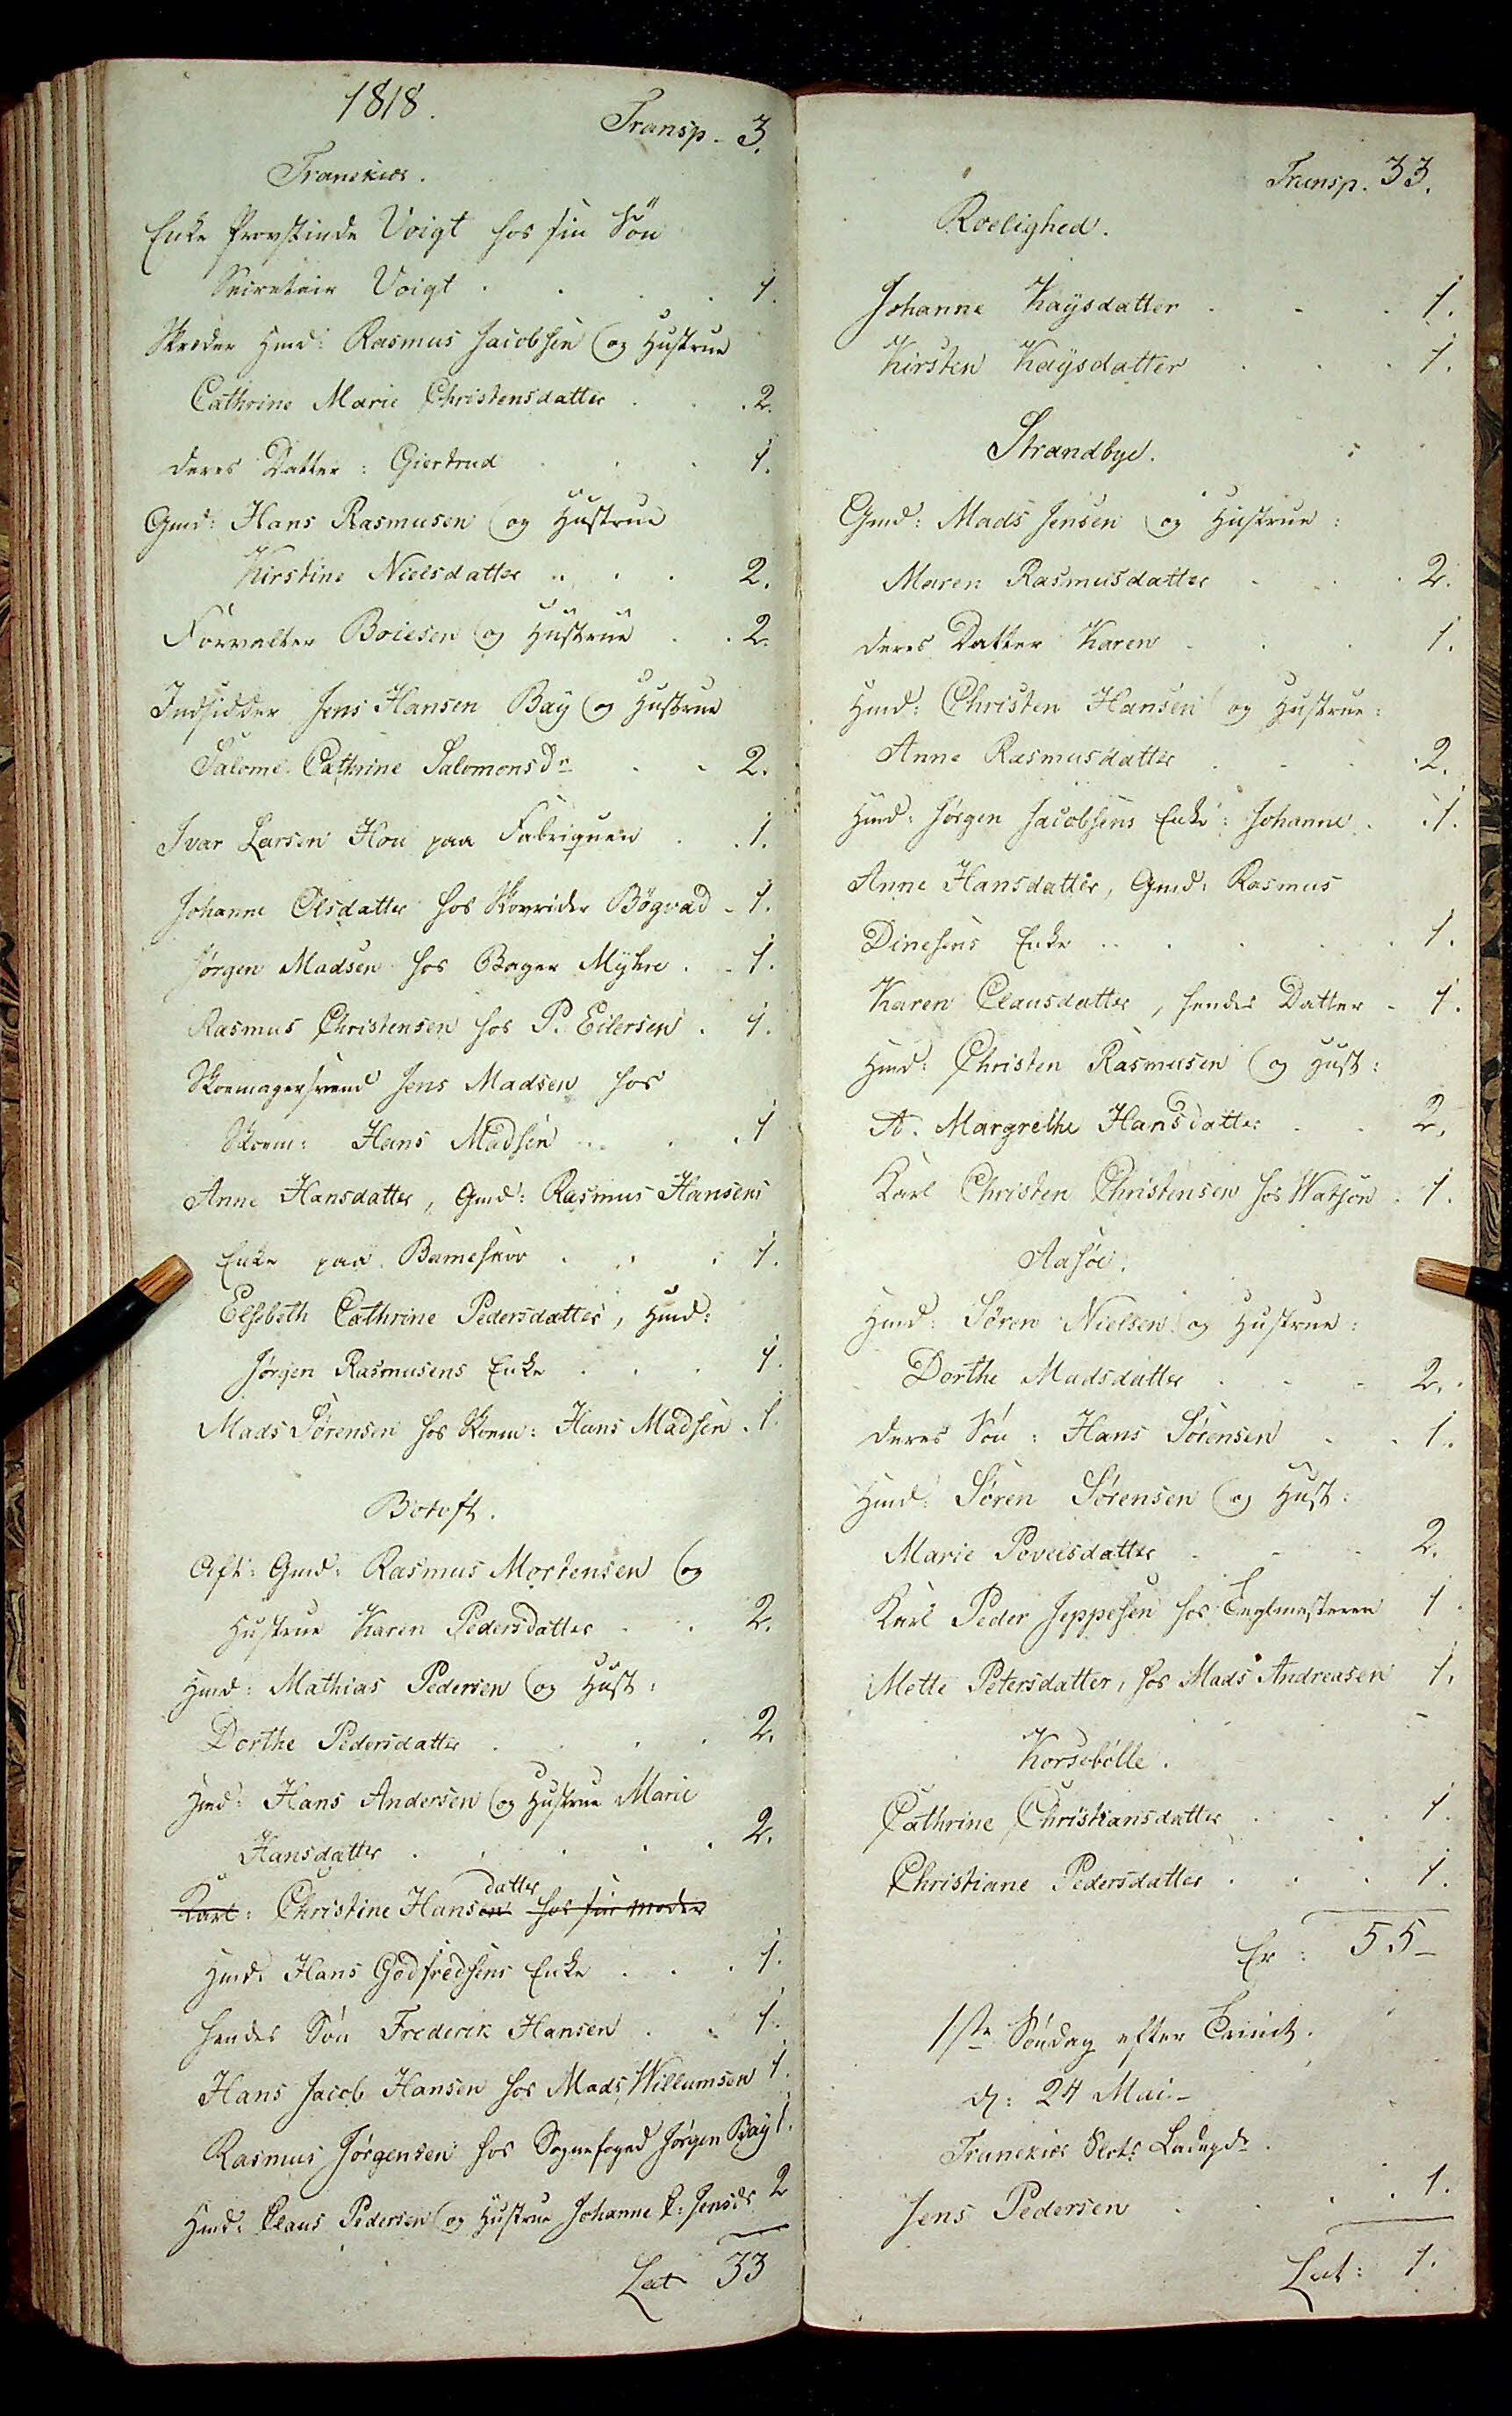1818
Transp - 3.
Tranekiær.
Enke Provstinde Voigt hos sin Søn
Secretair Voigt . . . . 1.
Skreder Hmd: Rasmus Jacobsen og Hustrue
Cathrine Marie Christensdatter . . . 2.
deres Datter: Giertrud . . . 1.
Gmd: Hans Rasmusen og Hustrue
Kirstine Nielsdatter . . . . 2.
Forvalter Boiesen og Hustrue . . 2.
Indsidder Jens Hansen Bay og Hustrue
Salome Cathrine Salomonsdr . .2.
Ivar Larsen Hou paa Fabriquen . . 1.
Johanne Olsdatter hos Skovrider Bøgvad - 1.
Jørgen Madsen hos Bagen Myhre . . 1.
Rasmus Christensen hos P. Eilersen . 1.
Skoemagersvend Jens Madsen hos
Skoem: Hans Madsen . . . 1
Anne Hansdatter, Gmd: Rasmus Hansens
Enke paa Bameskov . . . 1.
Elsebeth Cathrine Pedersdatter, Hmd:
Jørgen Rasmusens Enke . . . 1.
Mads Sørensen hos Skoem: Hans Madsen . 1.
Botoft.
Aft: Gmd: Rasmus Mortensen og
Hustrue Karen Pedersdatter . . 2.
Hmd: Mathias Pedersen og Hust:
Dorthe Pedersdatter . . . . 2.
Hmd: Hans Andersen og Hustrue Marie
Hansdatter . . . . . 2.
datter
Karl: Christine Hansen hos sin Morder
Hmd: Hans Godfredsens Enke . . . 1.
hendes Søn Frederik Hansen . . 1.
Hans Jacob Hansen hos Mads Willumsen 1.
Rasmus Jørgensen hos Sognefoged Jørgen Bay 1.
Hmd: Claus Pedersen og Hustrue Johanne C: Jensdr 2
Lat 33
Transp. 33.
Roelighed.
Johanne Kaysdatter . . . 1.
Kirsten Kaysdatter . . . 1.
Srrandbye.
Gmd: Mads Jensen og Hustrue:
Maren Rasmusdatter . . . 2.
deres Datter Karen . . . 1.
Hmd: Christen Hansen og Hustrue
Anne Rasmusdatter . . . . 2.
Hmd: Jørgen Jacobsens Enke: Johanne . . 1.
Anne Hansdatter, Gmd: Rasmus
Dinesens Enke . . . . . . 1.
Karen Clausdatter, hendes Datter . 1.
Hmd: Christen Rasmusen og Hust:
A. Margrethe Hansdatter . . 2.
Karl Christen Christensen hos Watson . 1.
Aasøe.
Hmd: Søren Nielsen og Hustrue:
Dorthe Madsdatter . . . 2.
deres Søn: Hans Sørensen . . 1.
Hmd: Søren Sørrensen og Hust:
Marie Povelsdatter . . . 2.
Karl Peder Jeppesen hos Teglmesteren 1.
Mette Pedersdatter, hos Hans Andreasen 1.
Korsebølle.
Cathrine Christiansdatter . . . 1.
Christiane Pedersdatter . . . 1.
Er: 55-
1ste Søndag efter Trinit.
d: 24 Mai-
Tranekiær Slots Ladegdr.
Jens Pedersen . . . . 1.
Lat: 1.
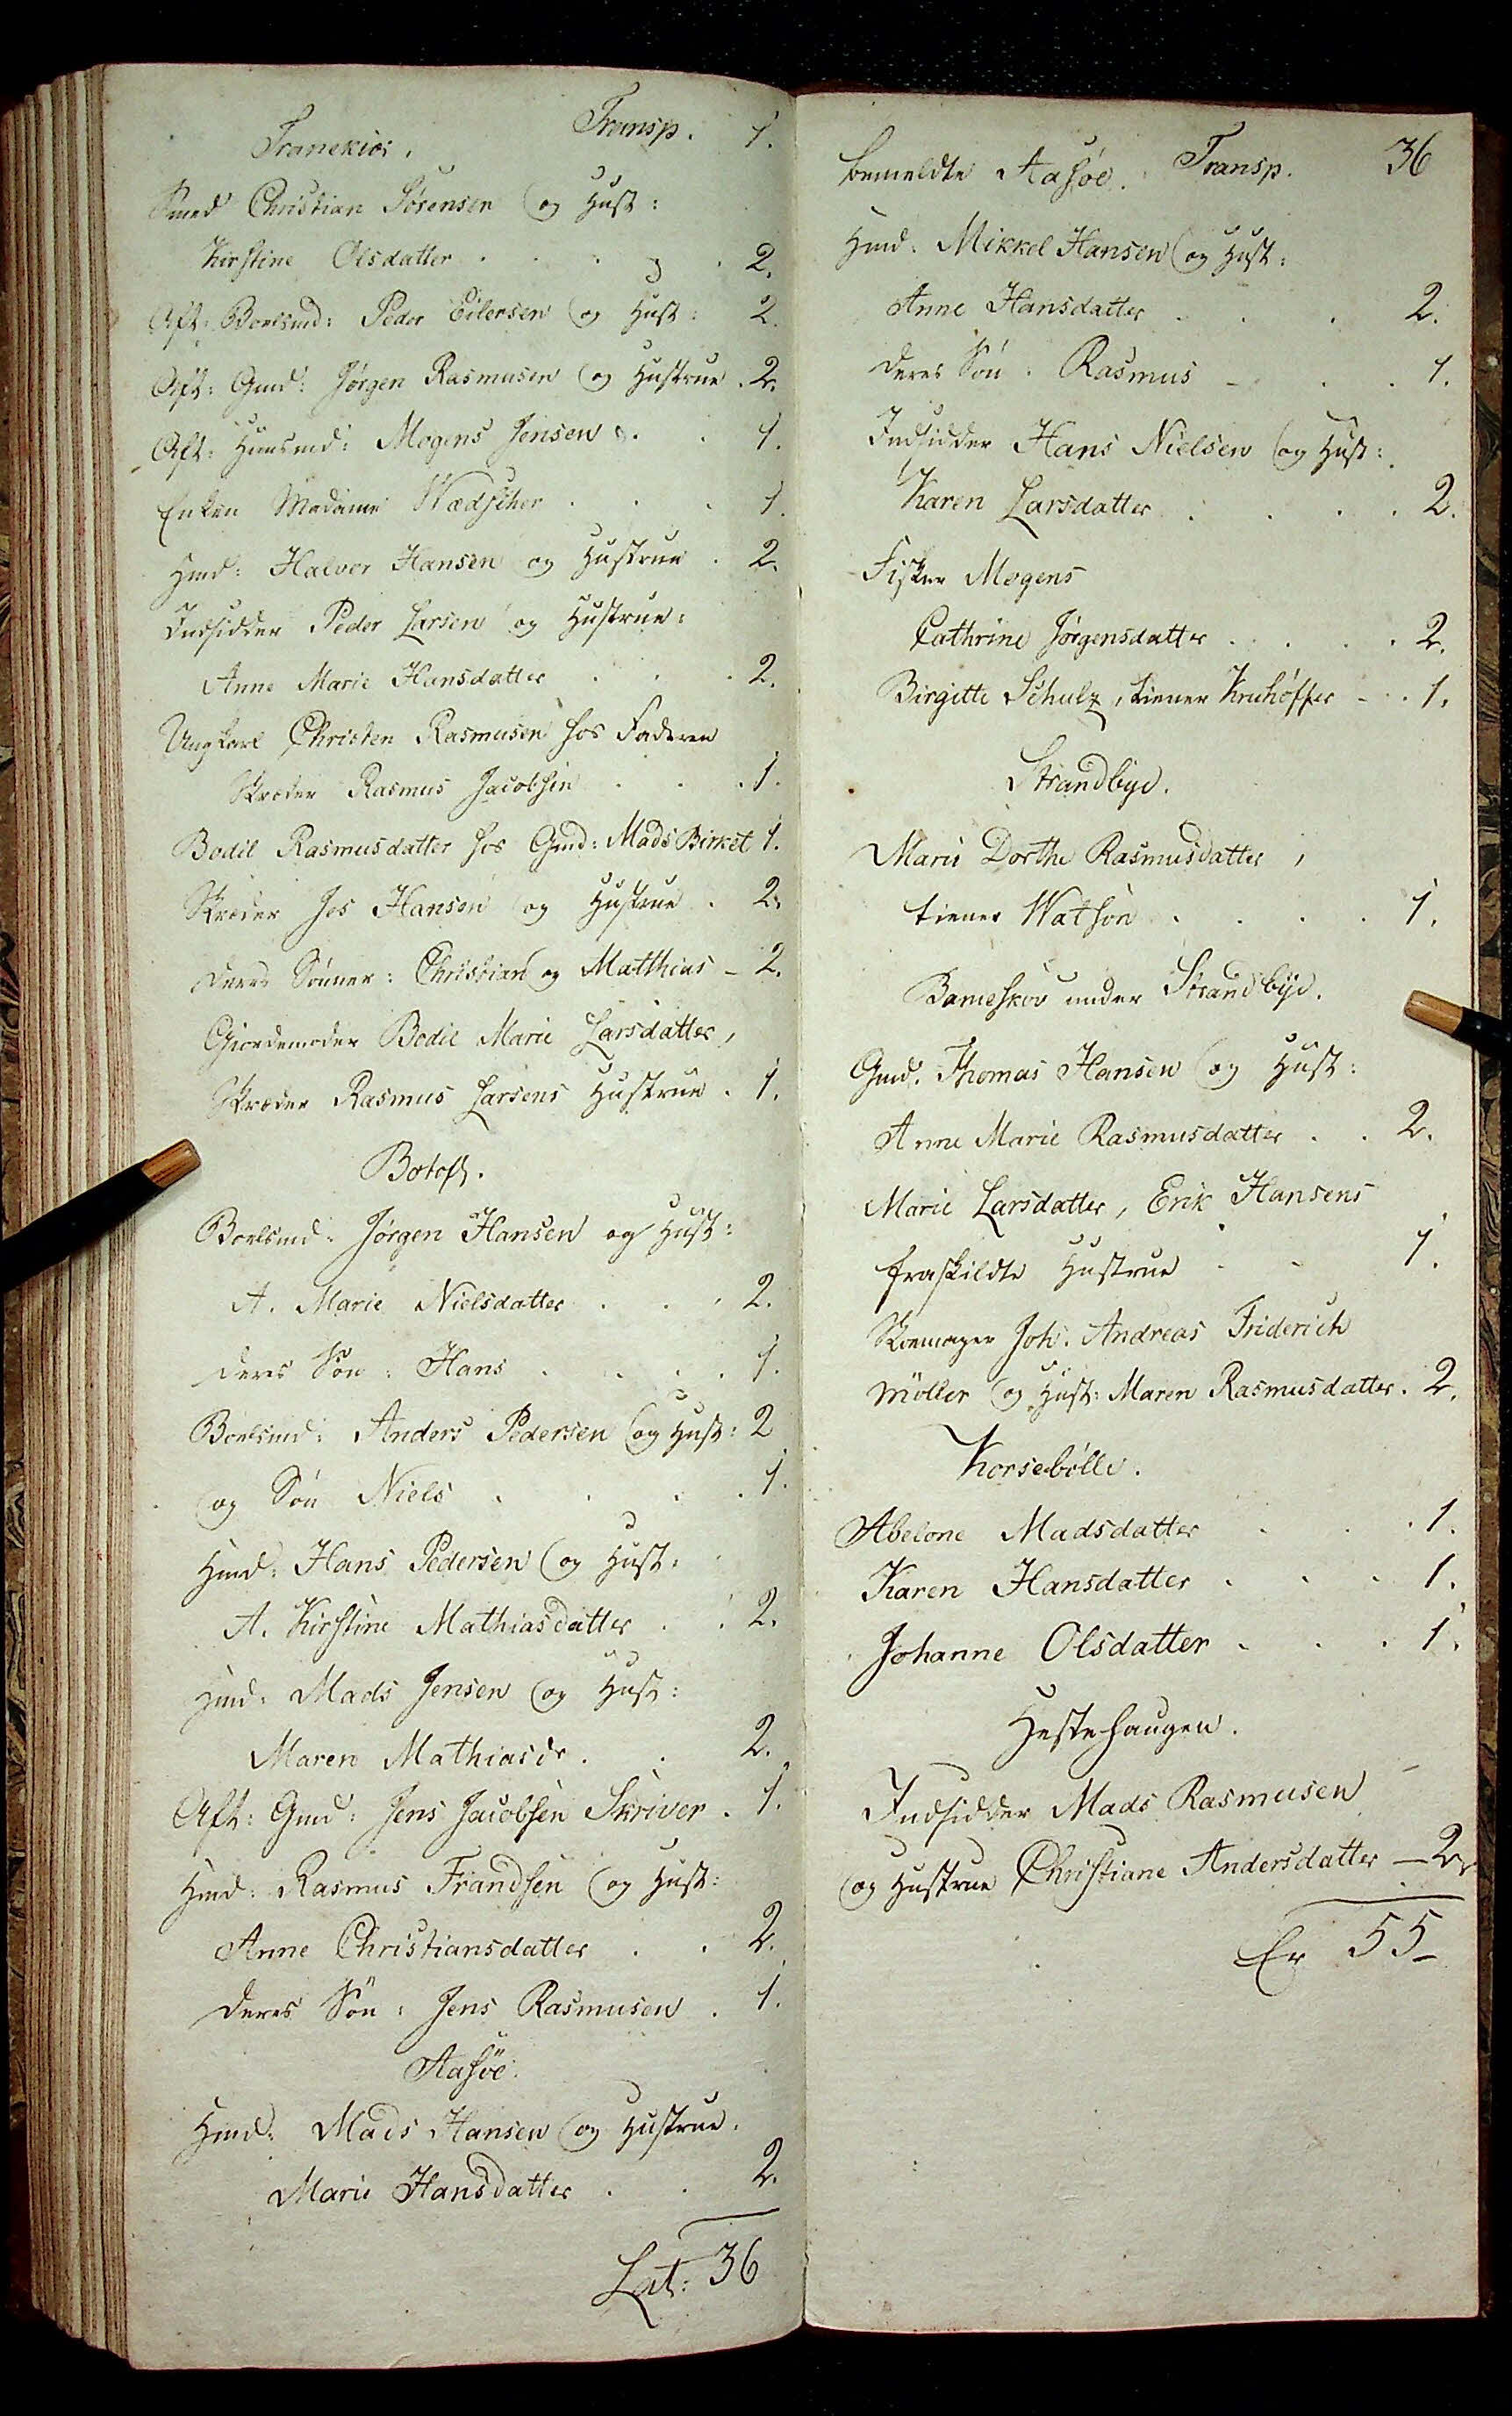Transp. 1.
Tranekiær.
Smed Christian Sørensen og Hust:
Kirstine Olsdatter . . . . . 2.
Aft: Boelsmd: Peder Eilersen og Hust: 2.
Aft: Gmd: Jørgen Rasmusen og Hustrue . 2.
Aft: Huusmd: Mogens Jensens . . . 1.
Enken Madame Wædscher . . . 1.
Hmd: Halver Hansen og Hustrue . 2.
Indsidder Peder Jensens og Hustrue:
Anne Marie Hansdatter . . . 2.
Ungkarl Christen Rasmusen hos Faderen
Skræder Rasmus Jacobsen . . . 1.
Bodil Rasmusdatter hos Gmd: Mads Birket 1.
Skræder Jes Hansen og Hustrue . 2.
deres Sønner: Christian og Matthias - 2.
Giordemoder Bodil Marie Larsdatter,
Skræder Rasmus Larsens Hustrue . 1.
Botoft.
Boelsmd: Jørgen Hansen og Hust:
A. Marie Nielsdatter . . . 2.
ders Søn: Hans . . . . 1.
Boelsmd: Anders Pedersen og Hust: 2
og Søn Niels . . . . 1.
Hmd: Hans Pedersen og Hust:
A. Kirstine Mathiasdatter . . 2.
Hmd: Mads Jensen og Hust:
Maren Mathiasdr . . 2.
Aft: Gmd: Jens Jacobsen Skriver . 1.
Hmd: Rasmus Frandsen og Hust:
Anne Christiansdatter . . 2.
deres Søn: Jens Rasmusen . 1.
Aasøe.
Hmd: Mads Hansen og Hustrue
Marie Hansdatter . . 2.
Lat: 36
Transp. 36
bemeldte Aasøe.
Hmd: Mikkel Hansen og Hustr:
Anne Hansdatter . . . 2.
deres Søn: Rasmus - . . . 1.
Indsidder Hans Nielsen og Hust:
Karen Larsdatter . . . . 2.
Fisker Mogens
Cathrine Jørgensdatter . . . . 2.
Birgitte Schulz, tiener Kruhøffer - . . 1.
Strandbye.
Marie Dorthe Rasmusdatter,
tiener Watson . . . . 1.
Bameskov under Strandbye.
Gmd. Thomas Hansen og Hust:
Anne Marie Rasmusdatter . . 2.
Marie Larsdatter, Erik Hansens
fraskildte Hustrue . . 1.
Skoemager Joh. Andreas Friderich
Møller og Hust: Maren Rasmusdatter . 2.
Korsebølle.
Abelone Madsdatter . . . 1.
Karen Hansdatter . . . 1.
Johanne Olsdatter . . . 1.
Hestehaugen.
Indsidder Mads Rasmusen
og Hustrue Christiane Andersdatter - 2.
Er 55-